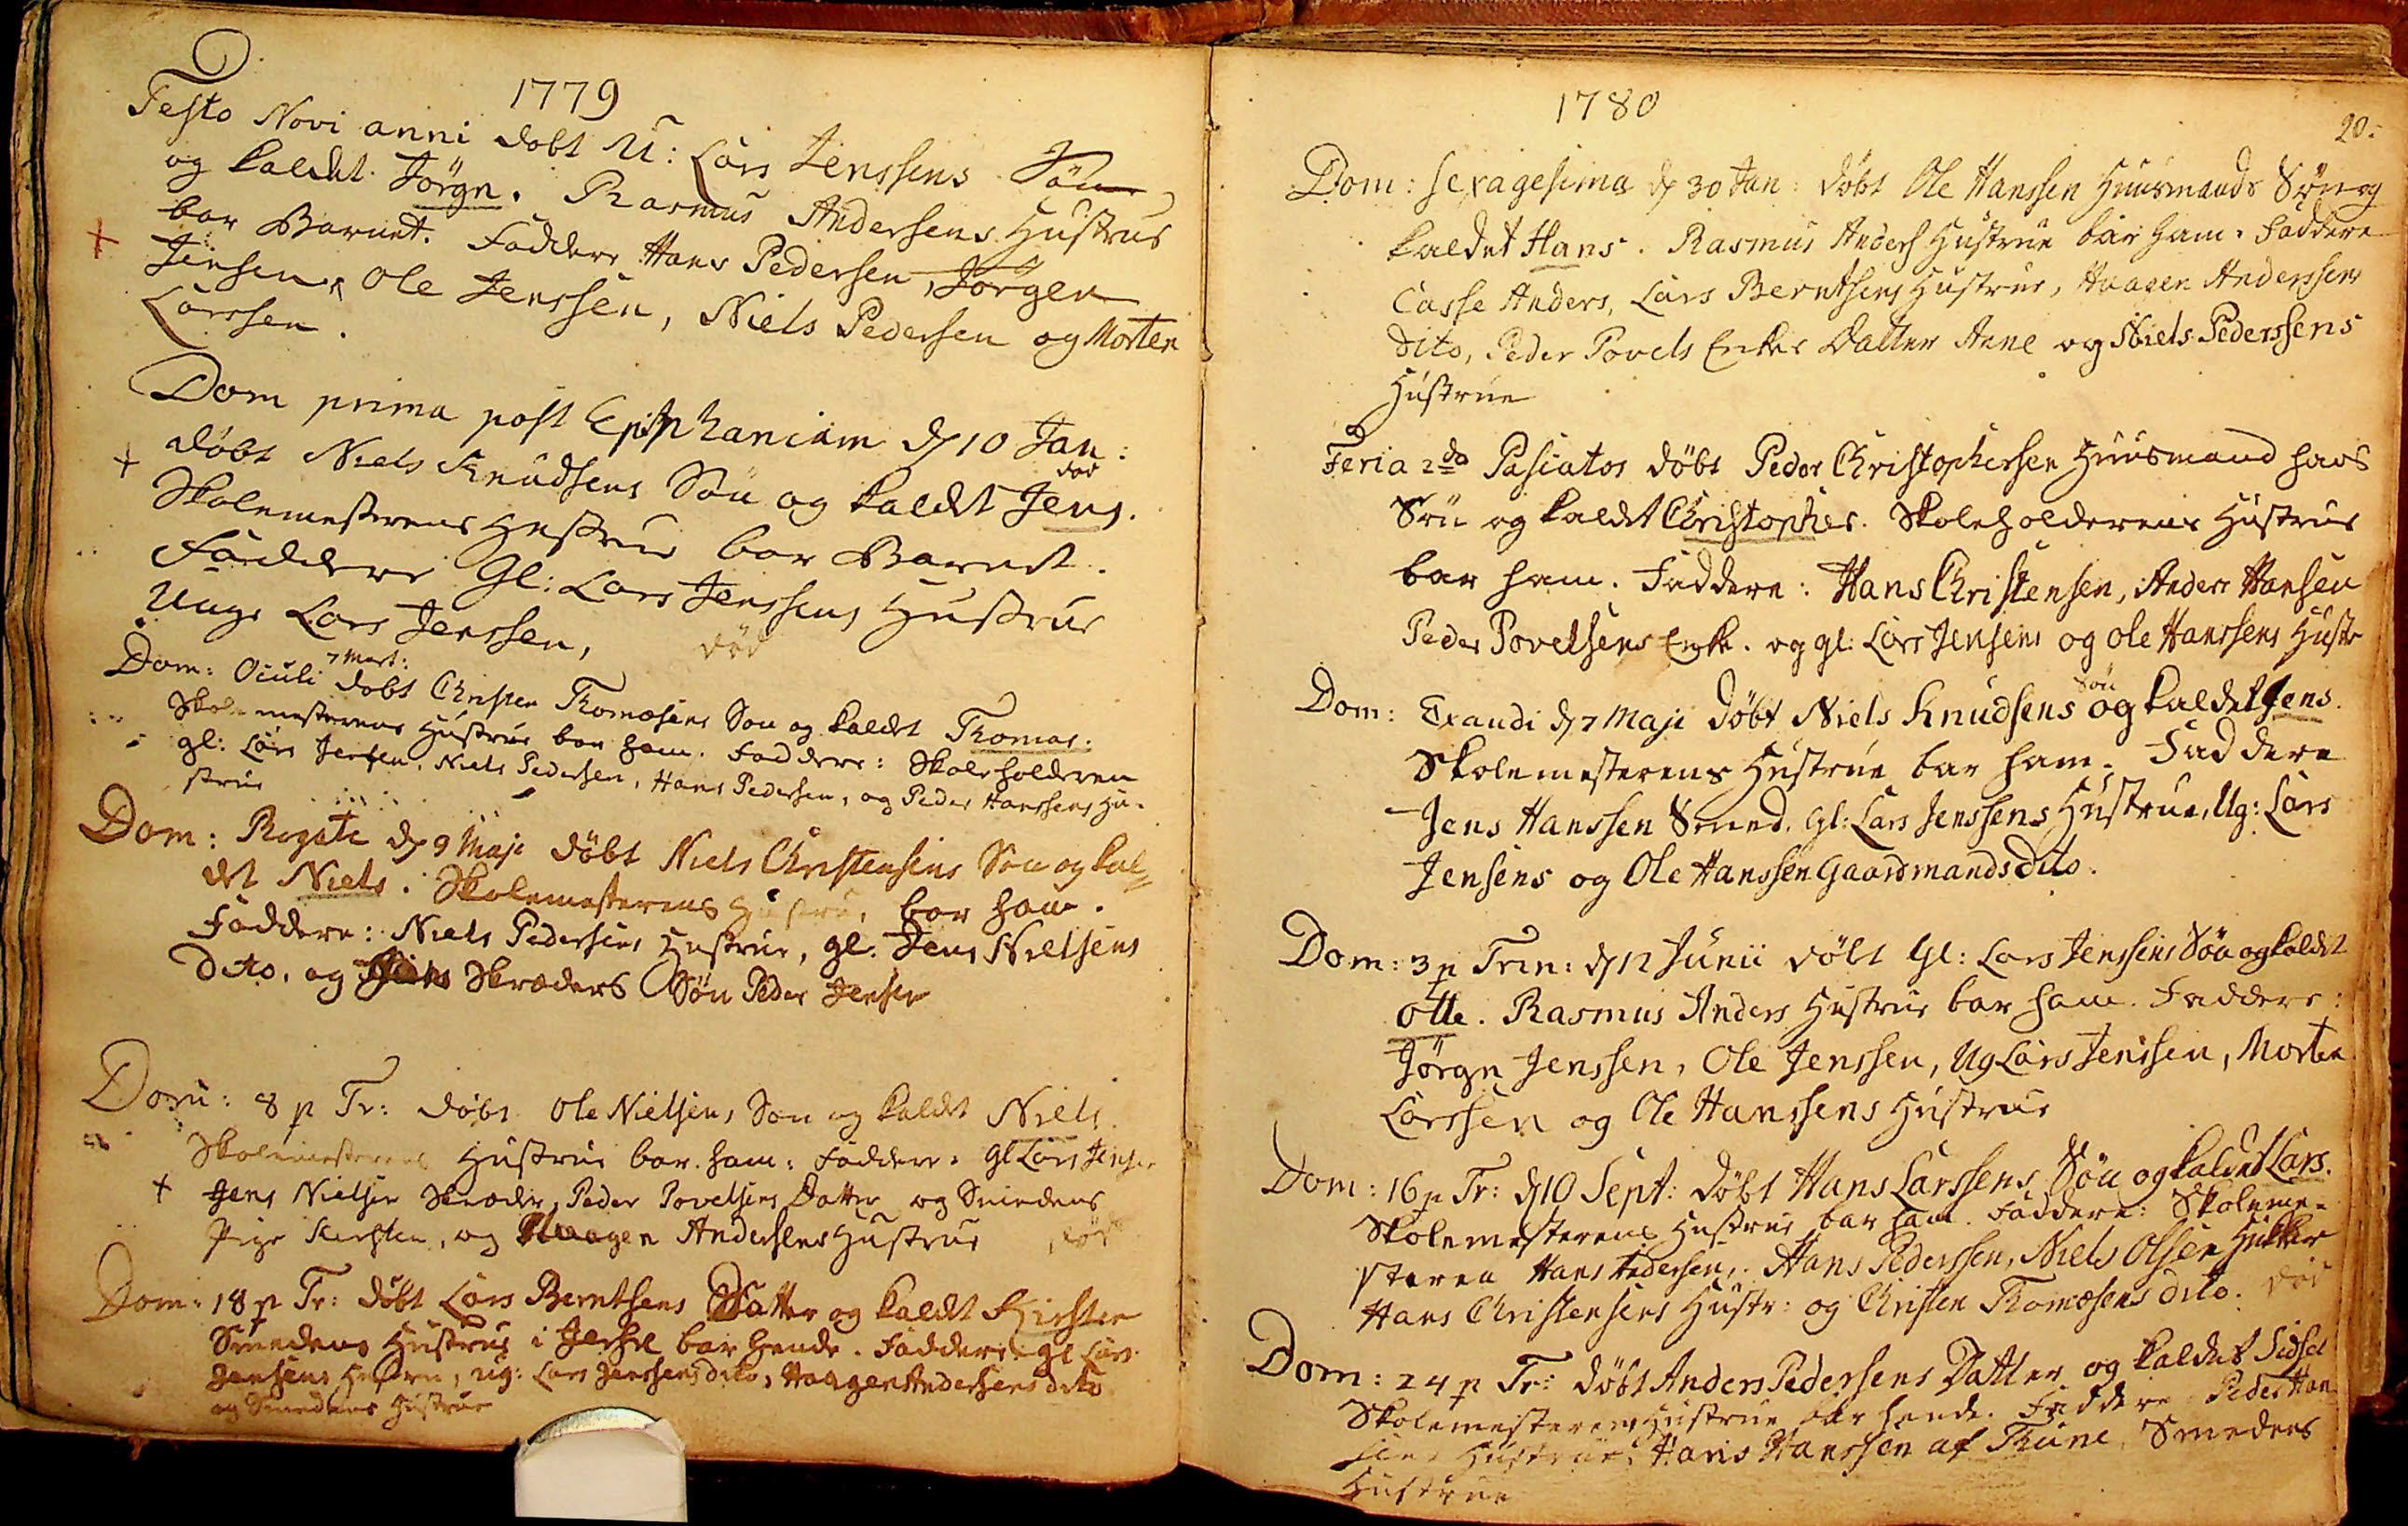1779
Festo Novi Anni døbt U: Lars Jenssens Søn
og kaldet Jørgn. Rasmus Andersens Hustrue
bar Barnet. Faddere Hans Pedersen, Jørgen
Jensen, Ole Jenssen, Niels Pedersen og Morten
Larssen.
Dom: prima post Epiphanium d 10 Jan:
død
døbt Niels Knudsens Søn og kaldet Jens.
Skolemesterens Hustrue bar Barnet.
Faddere Gl: Lars Jenssens Hustrue
Unge Lars Jenssen,
død
7 Mart:
Dom: Oculi døbt Christen Thomæsens Søn og Kaldet Thomas.
Skolemesterens Hustrue bar ham. Faddere: Skoleholderen
gl: Lars Jensen, Niels Pedersen, Hans Pedersen, og Peder Hanssens Hu¬
strue
Dom: Rogate d 9 Maji døbt Niels Christensens Søn og kal¬
det Niels. Skolemesterens Hustrue bar ham.
Faddere: Niels Pedersens Hustrue, gl. Jens Nielsens
dito, og Jens Skræders Søn Peder Jensen
Dom: 8 p Tr: døbt Ole Nielsens Søn og kaldet Niels.
Skolemesterens Hustrue bar ham: Faddere: gl Lars Jensen
Jens Nielsen Skræder, Peder Povelsens Datter og Smedens
Pige Kirsten, og Haagen Andersens Hustrue
død
Dom: 18 p Tr: døbt Lars Berntsens Datter og kaldet Kirsten
Smedens Hustrue i Jersie bar hende. Faddere: gl Lars
Jensens Hustrue, ug:Lars Jenssens dito,Haagen Andersens dito
og Smedens Hustrue
20.
1780
Dom: Sexagesima d 30 Jan: døbt Ole Hanssen Huusmands Søn og
kaldet Hans. Rasmus Anders. Hustrue bar ham. Faddere
Casse Anders, Lars Berntsens Hustrue, Haagen Anderssens
dito, Peder Povels Enkes Datter Anne og Niels Pederssens
Hustrue
Feria 2da Pascatos døbt Peder Christophersen Huusmand hans
Søn og kaldet Christopher. Skoleholderens Hustrue
bar ham. Faddere: Hans Christensen, Anders Hansen
Peder Povelsens Enke, og gl:Lars Jensens og Ole Hansens Hustr
Søn
Dom: Exaudi d 7 Maji døbt Niels Knudsens og kaldet Jens.
Skolemesterens Hustrue bar ham. Faddere
Jens Hanssen Smed, gl: Lars Jenssens Hustrue, Ug: Lars
Jensens og Ole Hanssen Gaardmands dito.
Dom: 3 p Trin: d 11 Junii døbt Gl: Lars Jenssens Søn og kaldet
Otte. Rasmus Anders Hustrue bar ham. Faddere:
Jørgn Jenssen, Ole Jenssen, Ug Lars Jenssen, Morten
Larssen og Ole Hanssens Hustrue
Dom: 16 p Tr: d 10 Sept: døbt Hans Larssens Søn og kaldet Lars.
Skolemesterens Hustrue bar ham. Faddere: Skoleme¬
steren Hans Andersen, Hans Pederssen, Niels Olsen Hukker
Hans Chistensens Hustr: og Christen Thomæsens dito. død
Dom: 24 p Tr: døbt Anders Pedersens Datter og kaldet Sidsel
Skolemesterens Hustrue bar hende. Faddere: Peder Han¬
sens Hustrue, Hans Hanssen af Thune, Smedens
Hustrue